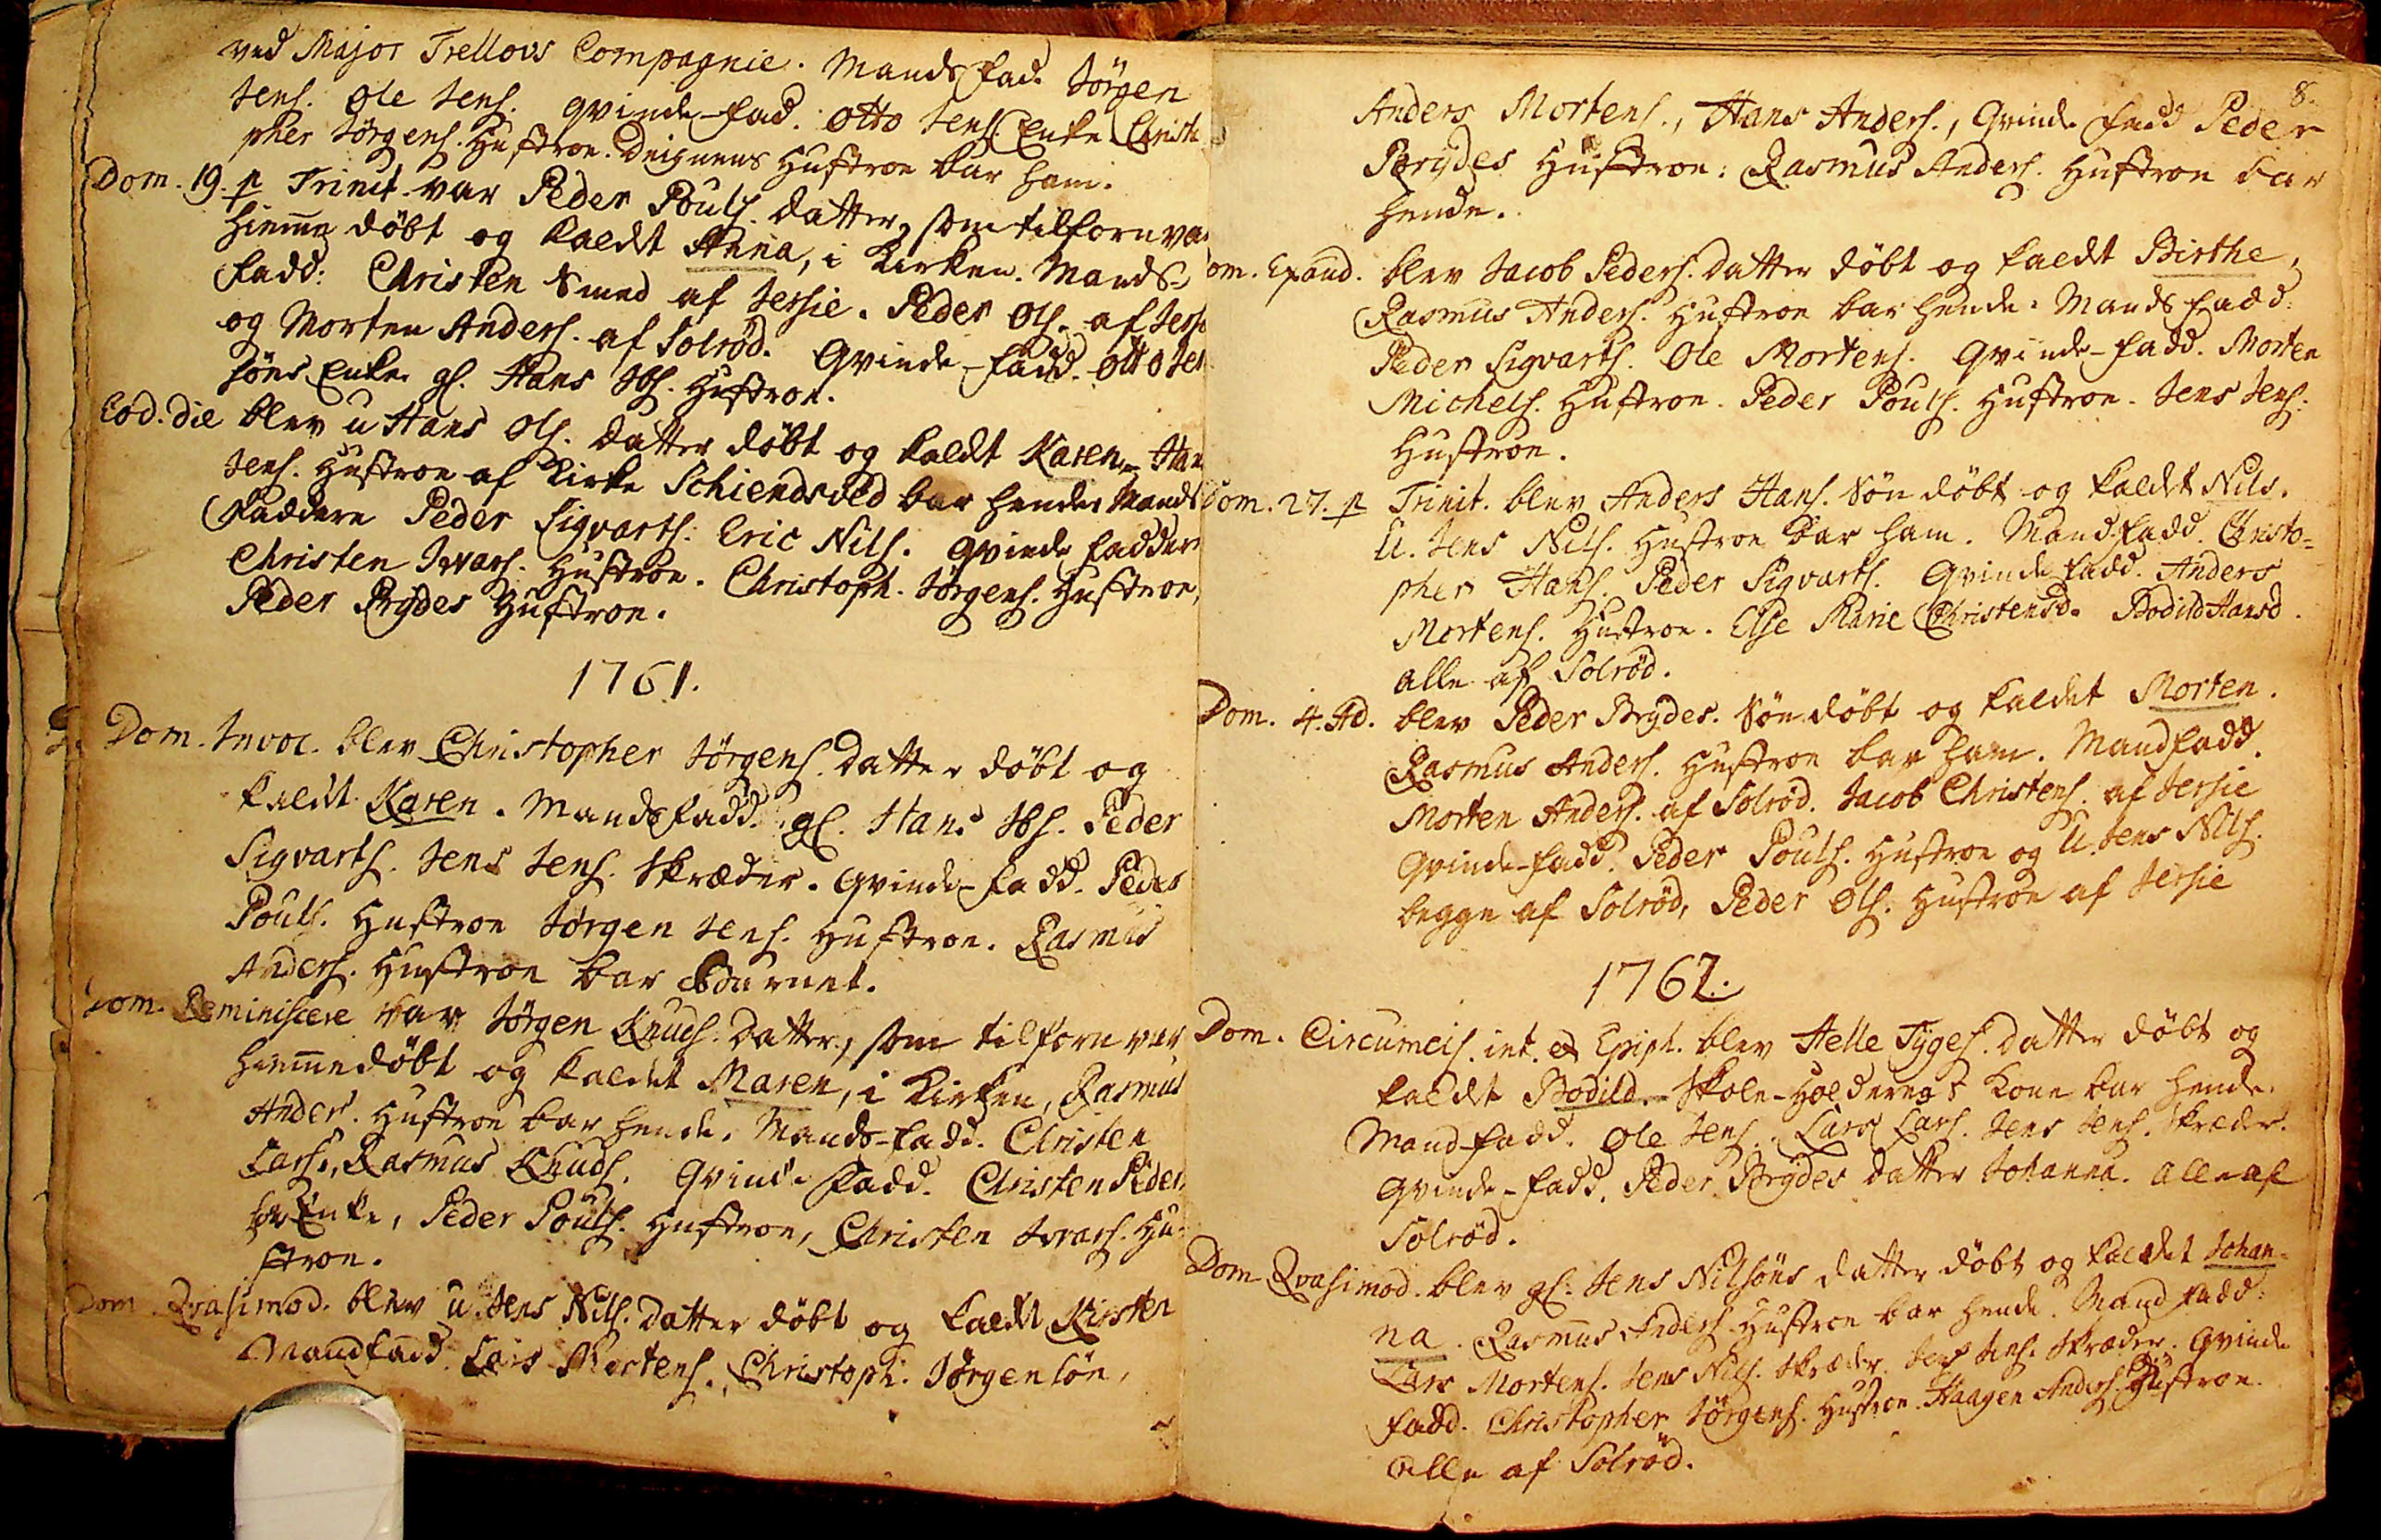ved Major Trellovs Compagnie. Mands fad. Jørgen
Jens. Ole Jens. qvinde-fad: Otto Jens. Enke Christo
pher Jørgens. Hustroe. Deignens Hustroe bar ham.
Dom. 19. p Trinit. var Peder Pouls. Datter, som tilforn var
hieme døbt og kaldt Anna, i Kirken. Mands¬
fadd: Christen Smed af Jersie. Peder Ols. af Jersie
Morten Anders. af Solrød. Qvinde-fadd. Otto Jen
søns Enke gl. Hans Ibs. Hustroe.
Eod. die blev u Hans Ols. Datter døbt og kaldt Karen Hans
Jens. Hustroe af Kirke Schiendsved bar hende, Mands
faddere Peder Sigvarts: Eric Nils. qvinde faddere
Christen Iwars. Hustroe. Christoph. Jørgens. Hustroe,
Peder Brydes Hustroe.
1761.
Dom: Ivoc. blev Christopher Jørgens. Datter døbt og
kaldet Karen. Mandsfadd. gl. Hans Ibs. Peder
Sigvarts. Jens Jens. Skræder. Qvinde fadd. Peder
Pouls Hustroe Jørgen Jens. Hustroe. Rasmus
Anders. Hustroe bar Barnet.
Dom. Reminiscere var Jørgen Knuds. Datter, som tilforn var
hiemedøbt og kaldet Maren, i Kirken, Rasmus
Anders: Hustroe bar hende. Mands-fadd. Christen
Lars., Rasmus Knuds. Qvinde-Fadd. Christen Peder¬
sens Enke, Peder Pouls. Hustroe, Christen Ivars. Hu¬
stroe.
Dom. Qvasimod: blev u. Jens Nils. Datter døbt og kaldet Kirsten
Mandfadd. Lars Mortens., Christoph. Jørgensøn,
8.
Anders Mortens., Hans Anders., Qvinde fadd Peder
Brydes Hustroe: Rasmus Anders. Hustroe bar
hende.
om. Exaud. blev Jacob Peders. Datter døbt og kaldet Birthe,
Rasmus Anders. Hustroe bar hende. Mands fadd:
Peder Sigvarts. Ole Mortens. Qvinde-fadd. Morten
Michels. Hustroe. Peder Pouls. Hustroe. Jens Jens:
Hustroe.
Dom. 27.p Trinit. blev Anders Hans. Søn døbt og kaldet Nils.
U. Jens Nils. Hustroe bar ham. Mand-fadd. Christo¬
pher Hans. Peder Sigvarts. Qvindefadd. Anders
Mortens. Hustroe. Else Marie Christensd. Bodild Hansd.
alle af Solrød.
Dom. 4.Ad. blev Peder Brydes. Søn døbt og kaldet Morten.
Rasmus Anders. Hustroe bar ham. Mandfadd.
Morten Anders. af Solrød. Jacob Christens. af Jersie
Qvinde-fadd. Peder Pouls. Hustroe og U. Jens Nils.
begge af Solrød, Peder Ols: Hustroe af Jersie.
1762.
Dom. Circumcis. int. ex Epiph. blev Helle Tyges. Datter døbt og
kaldet Bodild. Skole-Holderens Kone bar hende.
Mand-fadd. Ole Jens. Lars Lars. Jens Jens. Skræder.
Qvinde-fadd. Peder Brydes Datter Johanna. Alle af
Solrød.
Dom Qvasimod. blev gl. Jens Nielsøns datter døbt og kaldet Johan¬
na. Rasmus Anders. Hustroe bar hende. Mand-fadd:
Lars Mortens. Jens Nils Skræder. Jens Jens Skræder. Qvinde
fadd. Christopher Jørgens. Hustroe Haagen Anders. Hustroe.
Alle af Solrød.
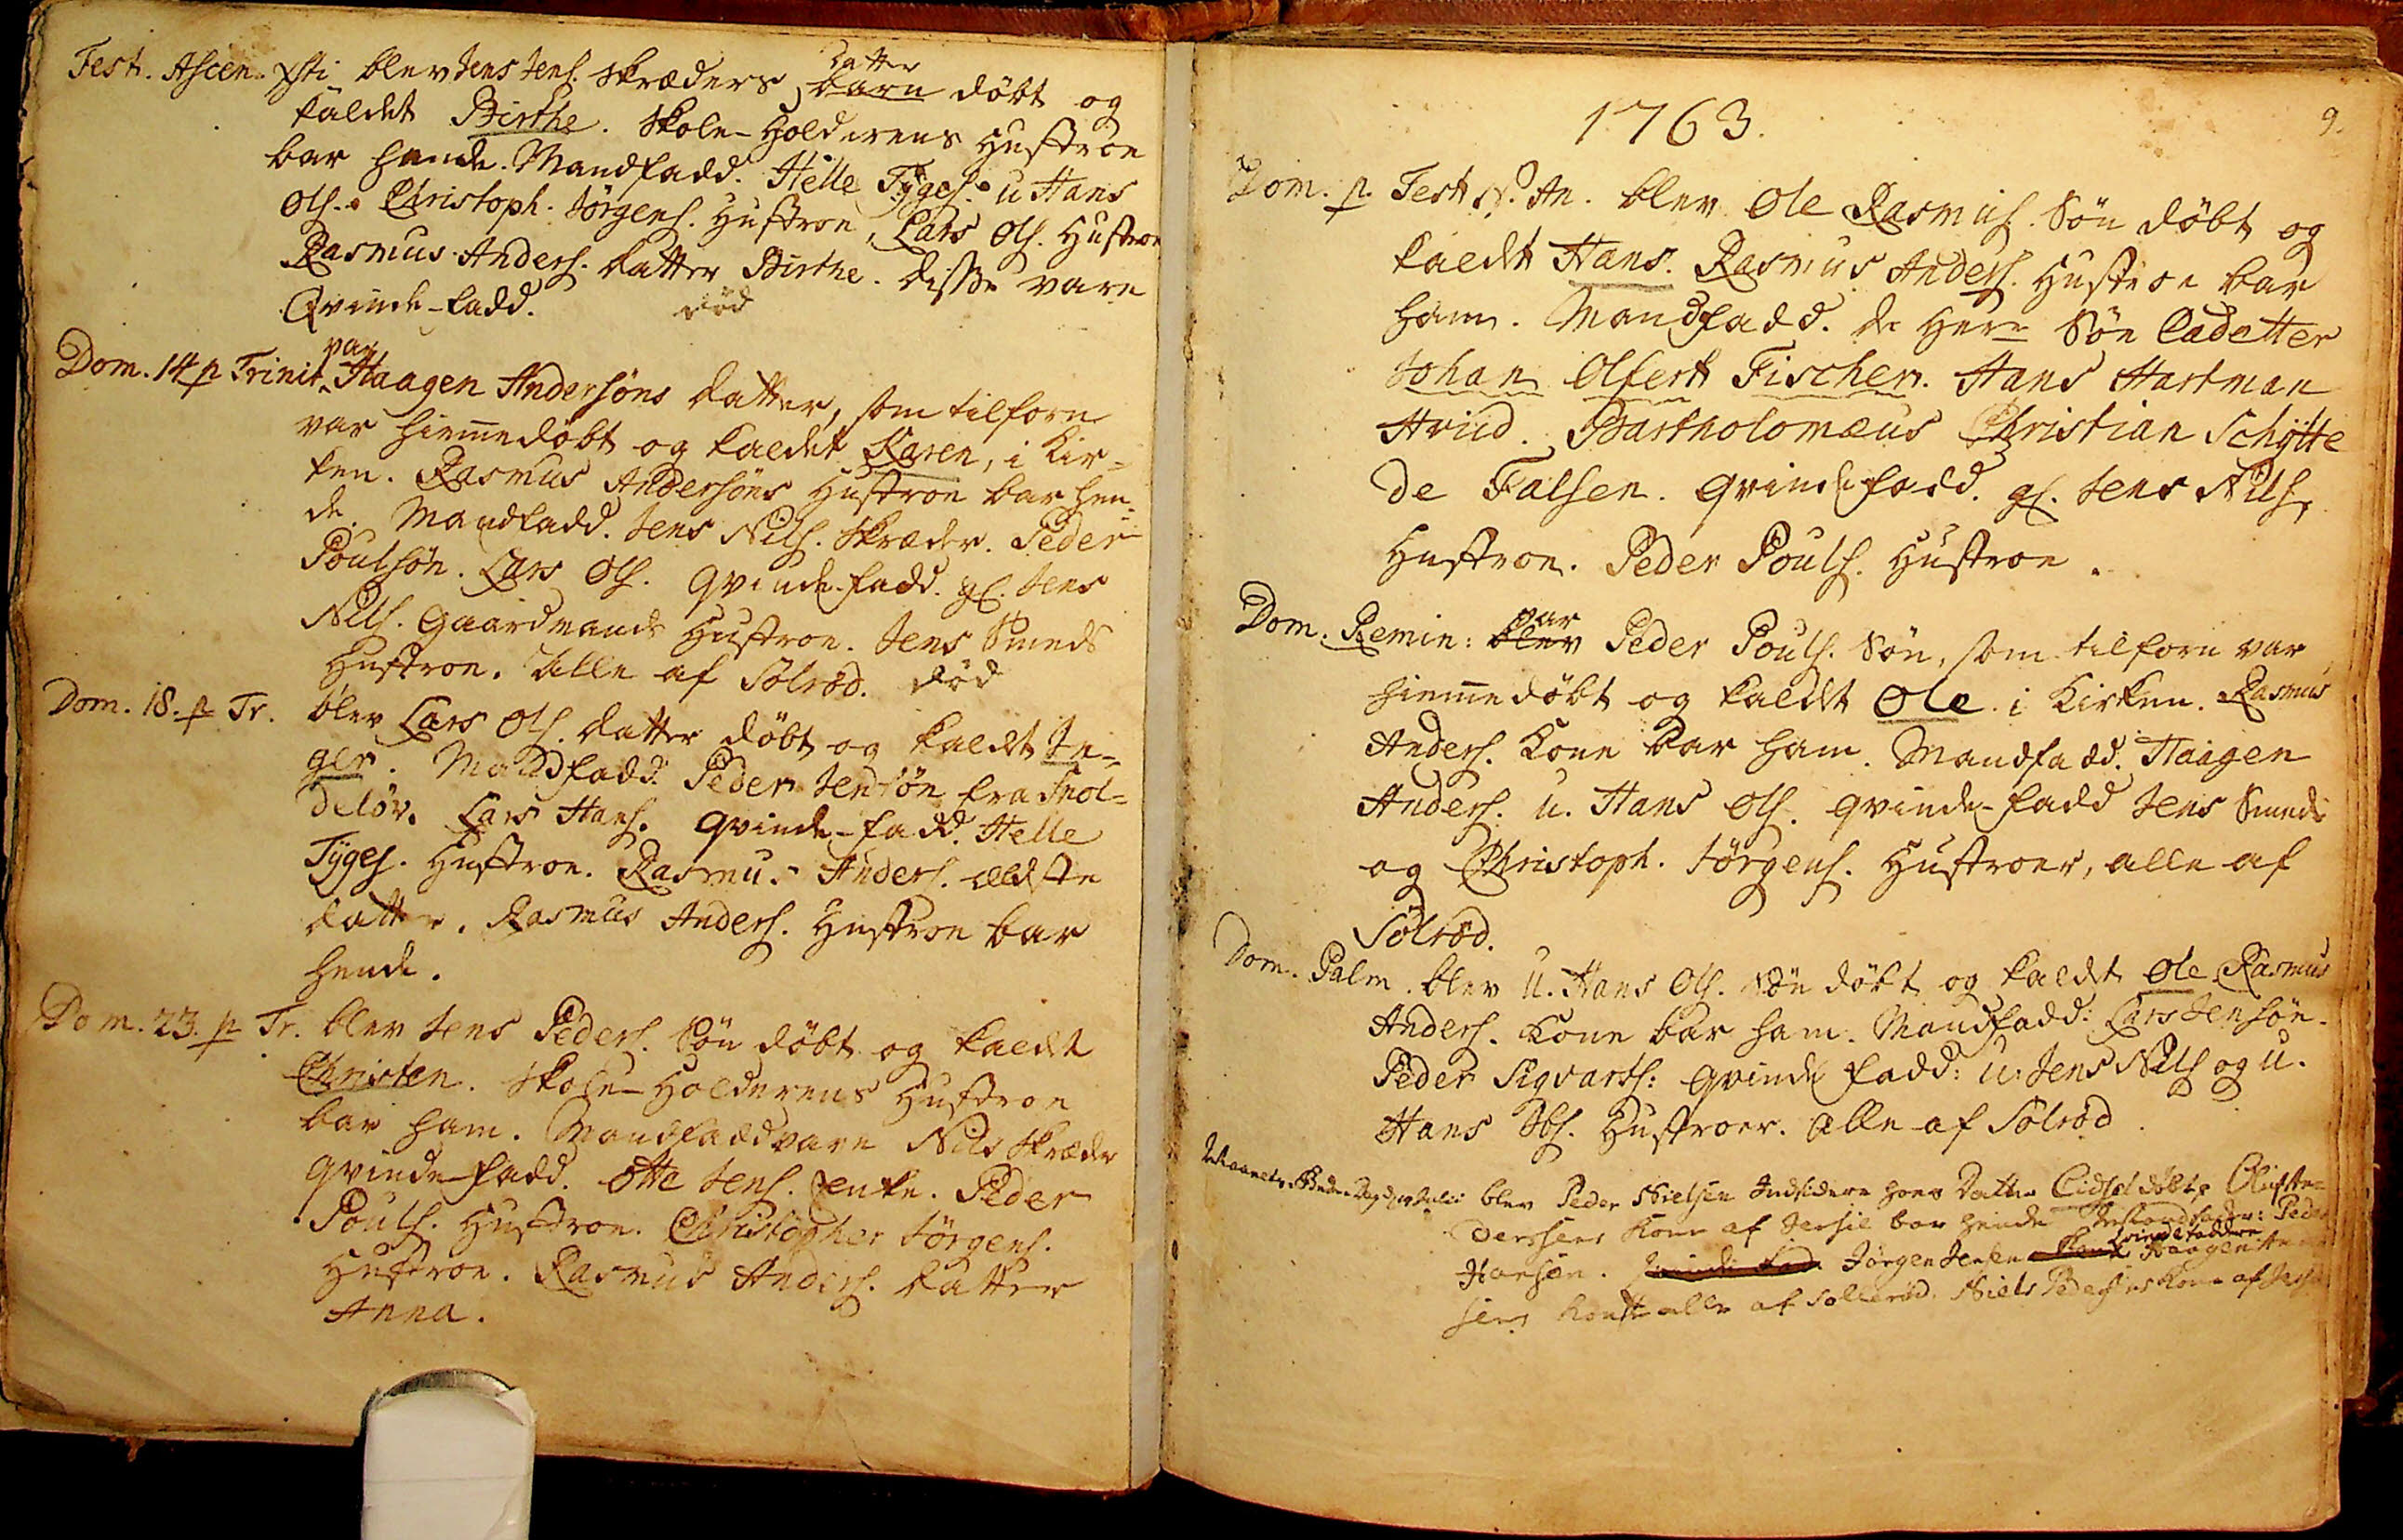Datter
Fest. Ascen. xsti blev Jens Jens. skræders barn døbt og
kaldet Birthe. Skole-Holderens Hustroe
bar hende. Mandfadd. Helle Tyges: u Hans
Ols. Christoph. Jørgens. Hustroe, Lars Ols. Hustroe
Rasmus Anders. Datter Birthe. Disse vare
Qvinde-fadd.
død
var
Dom. 14 p Trinit Haagen Andersøns Datter, som tilforn
var hiemedøbt og kaldet Karen, i Kir¬
ken. Rasmus Andersøns Hustroe bar hen¬
de. Mandfadd. Jens Nils. Skræder. Peder
Poulsøn. Lars Ols. Qvinde-fadd. gl. Jens
Nils. Gaardmands Hustrue. Jens Smeds
Hustroe. Alle af Solrød. død
Dom. 18. p Tr. blev Lars Ols. Datter døbt og kaldet In¬
ger. Mandsfadd. Peder Jensøn fra Snol¬
deløv. Lars Hans. Qvinde-fadd. Helle
Tyges. Hustroe. Rasmus Anders. ældste
Datter. Rasmus Anders. Hustroe bar
hende.
Dom. 23. p Tr. Jens Peders. Søn døbt og kaldet
Christen. Skole-Holderens Hustroe
bar ham. Mandfadd vare Nils Skræder
Qvinde-fadd. Otte Jens. Enke. Peder
Pouls. Hustroe. Christopher Jørgens.
Hustroe. Rasmus Anders. Datter
Anna.
9.
1763.
Dom. p. Fest N. An. blev Ole Rasmus. Søn døbt og
kaldt Hans Rasmus Anders. Hustroe bar
ham. Mandfadd. De Herr Søe Cadetter
Johan Olfert Fischer. Hans Hartman
Hvid. Bartholomæus Christian Schytte
de Falsen. Qvindefadd. gl. Jens Niels.
Hustroe. Peder Pouls. Hustroe.
var
Dom. Remin: blev Peder Pouls. Søn, som tilforn var
hiemedøbt og kaldt Ole, i Kirken. Rasmus
Anders. Kone bar ham. Mandfadd. Haagen
Anders. u. Hans Ols. Qvinde-fadd. Jens Smeds
og Christoph. Jørgens. Hustroer, alle af
Solrød.
Dom. Palm. blev U. Hans Ols. Søn døbt og kaldt Ole. Rasmus
Anders. Kone bar ham. Mandfadd: Lars Jensøn.
Peder Sigvarts: Qvinde fadd: U: Jens Nils og U.
Hans Ibs. Hustroer. Alle af Solrød.
Maanets=Bede=Dag d 1## Julii blev Peder Nielsen Indsidere hans Datter Cidsel døbt. Oluf An¬
dersens Kone af Jersie bar hende Mandfadder: Peder
Qvindefaddere
Hansen. Qvinde fad. Jørgen Jensens Kone Haagen Ander
sens Kone alle af Sollerød. Niels Pedersens Kone af Jersie